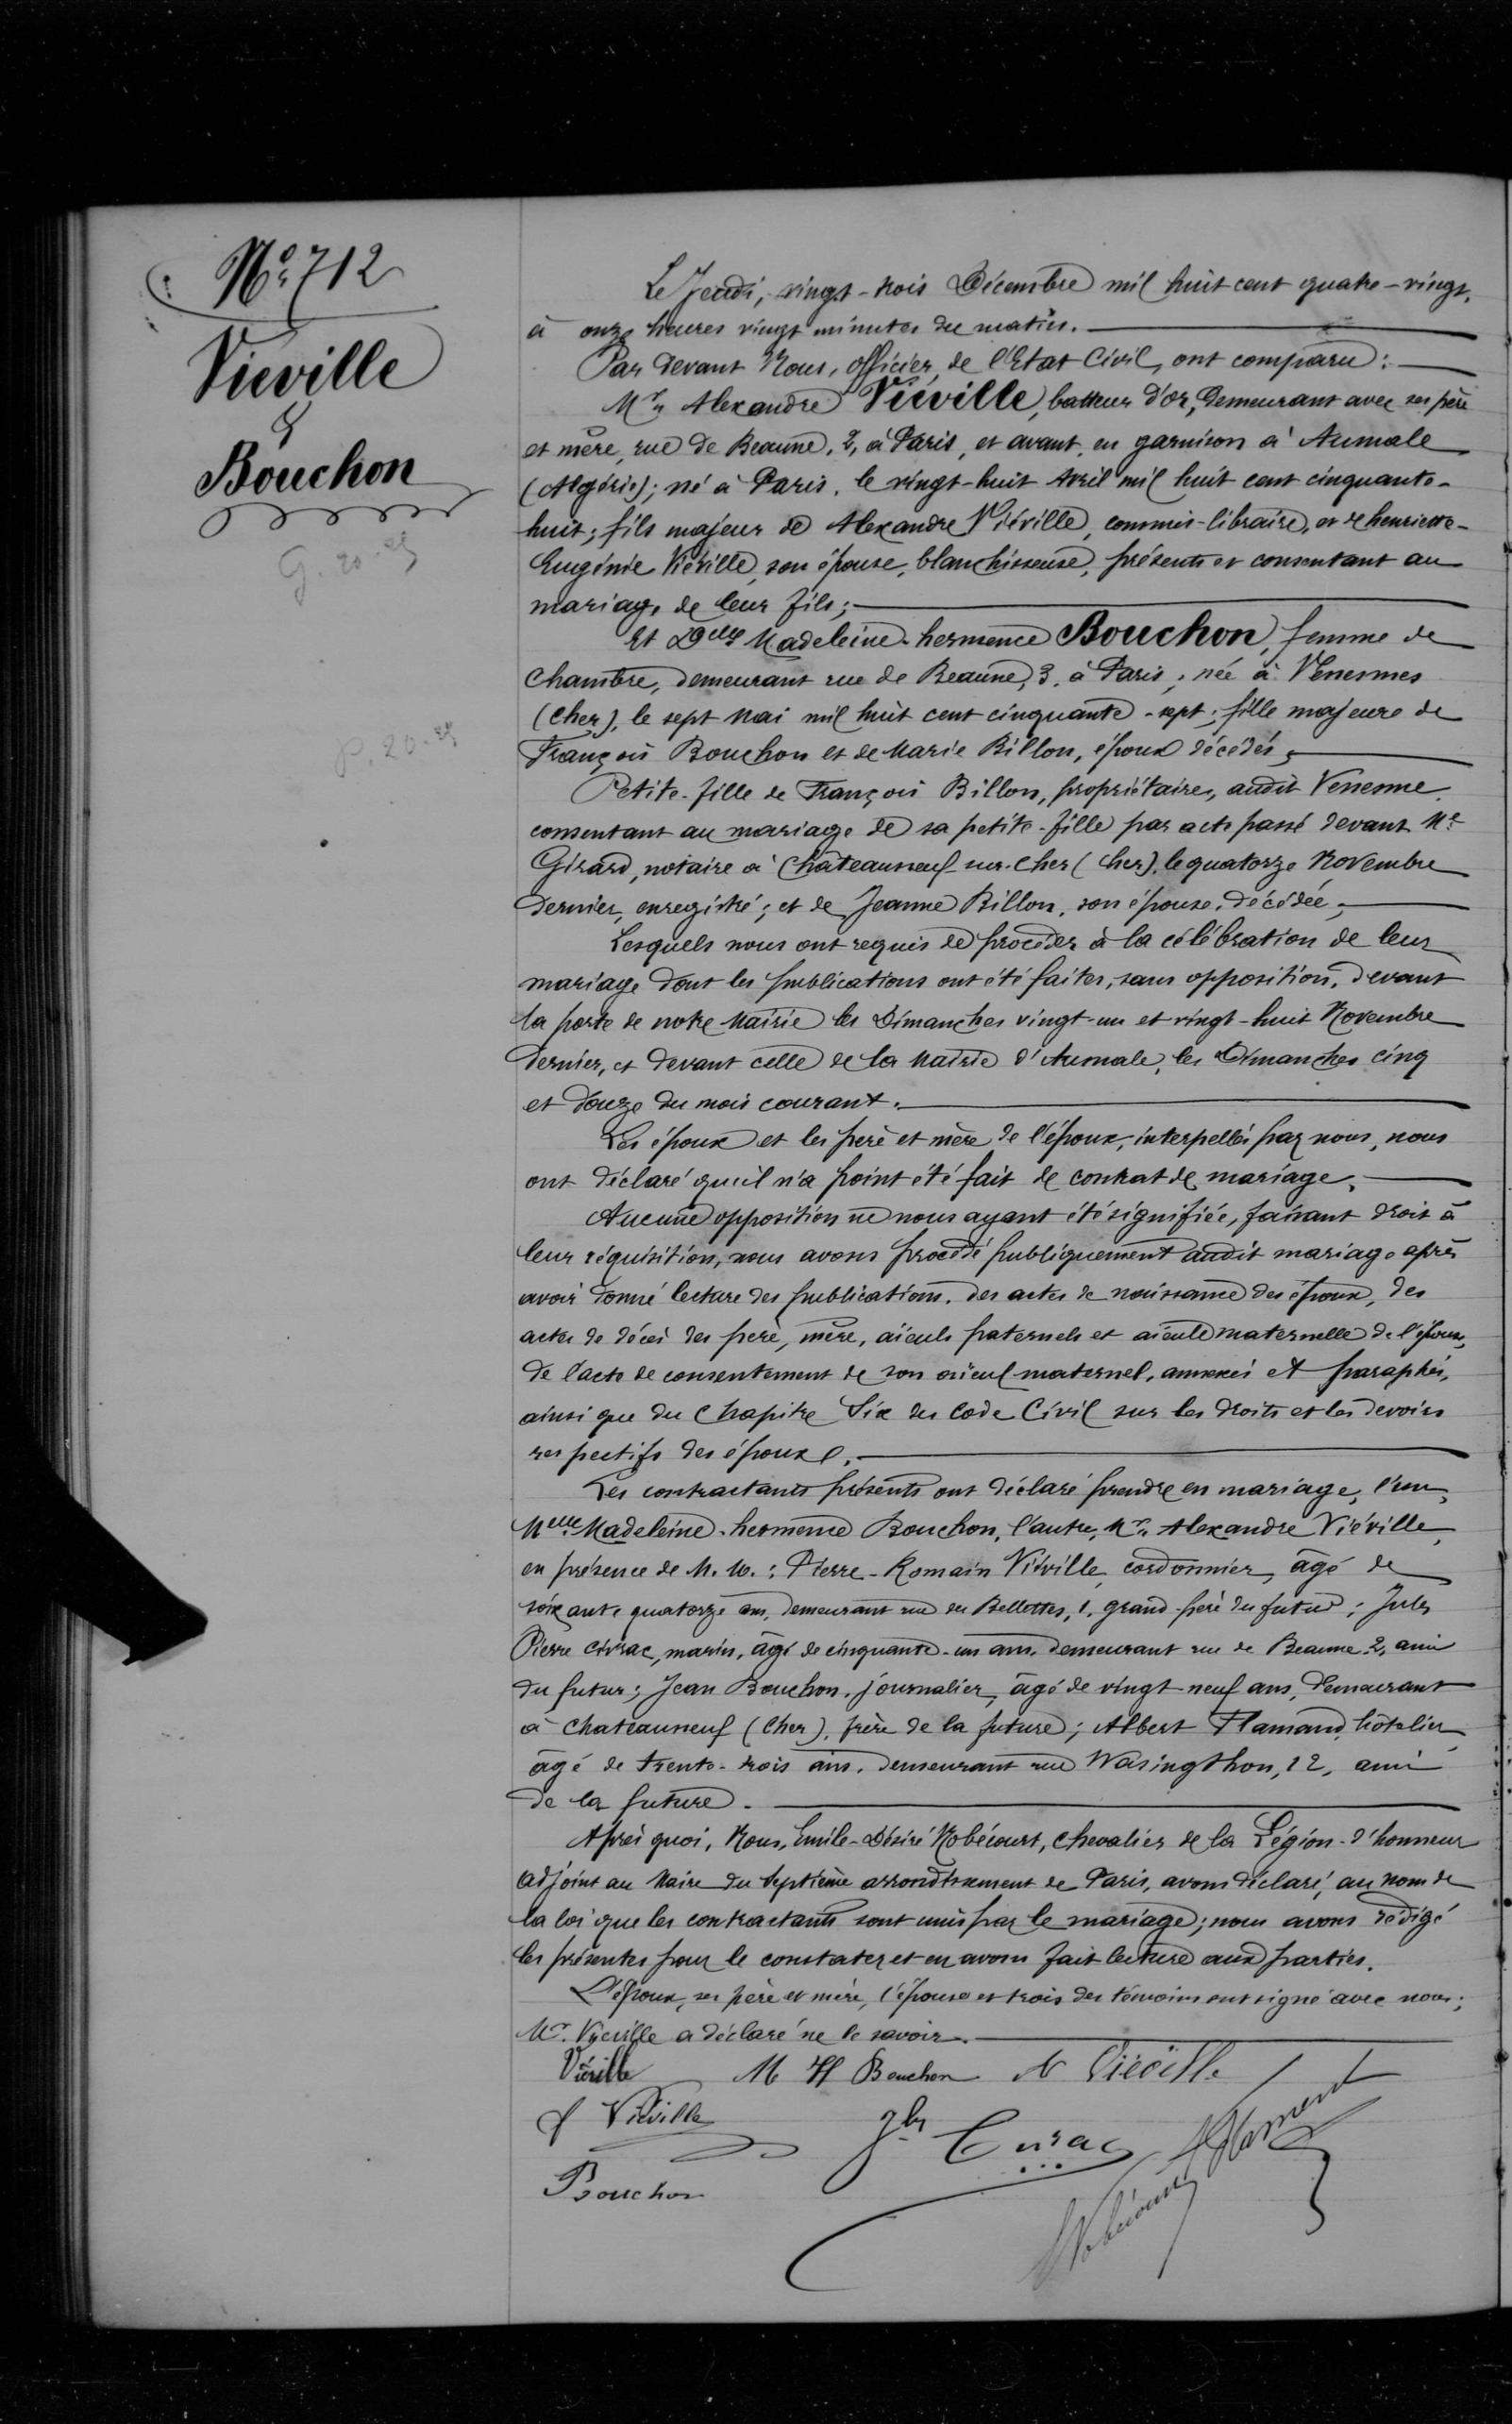N°712
Vieville
&
Bouchon
Le Jeudi, vingt-trois Décémbre mil huit cent quatre-vingt,
à onze heures vingt minutes du matin.
Par Devant Nous, officier de l'Etat Civil, ont comparu:
Me Alexandre Vieville, batteur d'or, demeurant avec ses père
et mère, rue de Beaume, 2, à Paris, et avant, en garnison à Aumale
(Algérie); né à Paris, le vingt-huit Avril mil huit cent cinquante-
huit; fils majeur de Alexandre Vieville, commis-libraire, et de Henriette-
Eugénie Vieville, son épouse, blanchisseuse, présente et consentant au
mariage, de leur fils;
Et Delle Madeleine Hermence Bouchon, femme de
chambre, demeurant rue de Beaume, 3, à Paris; née à Venesmes
(Cher), le sept mai mil huit cent cinquante-sept; fille majeure de
François Bouchon et de Marie Billon, époux décédés
Petite-fille de François Billon, propriétaire, audit Venesme
consentant au mariage de sa petite-fille par acte passé devant Me
Girard, notaire à Chateauneuf-sur-Cher (Cher) le quatorze novembre
dernier, enregistré; et de Jeanne Billon, son épouse, décédée,
Lesquels nous ont requis de procéder à la célébration de leur
mariage dont les publications ont été faites sans opposition, devant
sa porte de notre mairie les Dimanches vingt un et vingt huit novembre
dernier, et devant celle de la Mairie d'Aumale, les Dimanches cinq
et douze des mois courant.
Les époux et les père et mère de l'époux, interpellés par nous, nous
ont déclaré qu'il n'a point été fait de contrat de mariage.
Aucune opposition ne nous ayant été signifiée, faisant droit à
leur réquisition, nous avons procédé publiquement audit mariage après
avoir donné lecture des publications des actes de naissance des époux, des
actes de décès des père, mère, aïeuls paternels et aieule maternelle de l'épouse
de l'acte de consentement de son aïeu maternel, annexés et paraphés,
ainsi que du Chapitre six des Code Civil sur les droits et les devoirs
respectifs des époux.
Les contractants présents ont déclaré prendre en mariage, l'un,
Melle Madeleine Hermeme Bouchon, l'autre Mr Alexandre Viéville
en présence de M.M: Pierre Romain Viéville, cordonnier, âgé de
soixante quatorze ans, demeurant rue des Bellettes, 1, grand-frère des futurs: Jules
Pierre Cussac, marin, âgé de cinquante-un an, demeurant rue de Beaume, 2, ami
du futur; Jean Bouchon, journalier, âgé de vingt-neuf ans, demeurant
à Chateauneuf (Cher), père de la future; Albert Flamand hôtelier
âgé de trente-trois ans, demeurant rue Wasingthon, 22, ami
de la future.
Après quoi, Nous, Emile-Désirée Nobécourt, Chevalier de la Légion d'honneur
adjoint au Maire du septième arrondissement de Paris, avons déclaré, au nom de
la loi que les contractants sont unis par le mariage; nous avons rédigé
les présenter pour le constater et nous avons fait lecture aux parties.
L'époux, son père et mère, l'épouse et trois des témoins ont signé avec nous,
Me Vieville a déclaré ne le savoir.
Viéville M ll Bouchon M Viéville
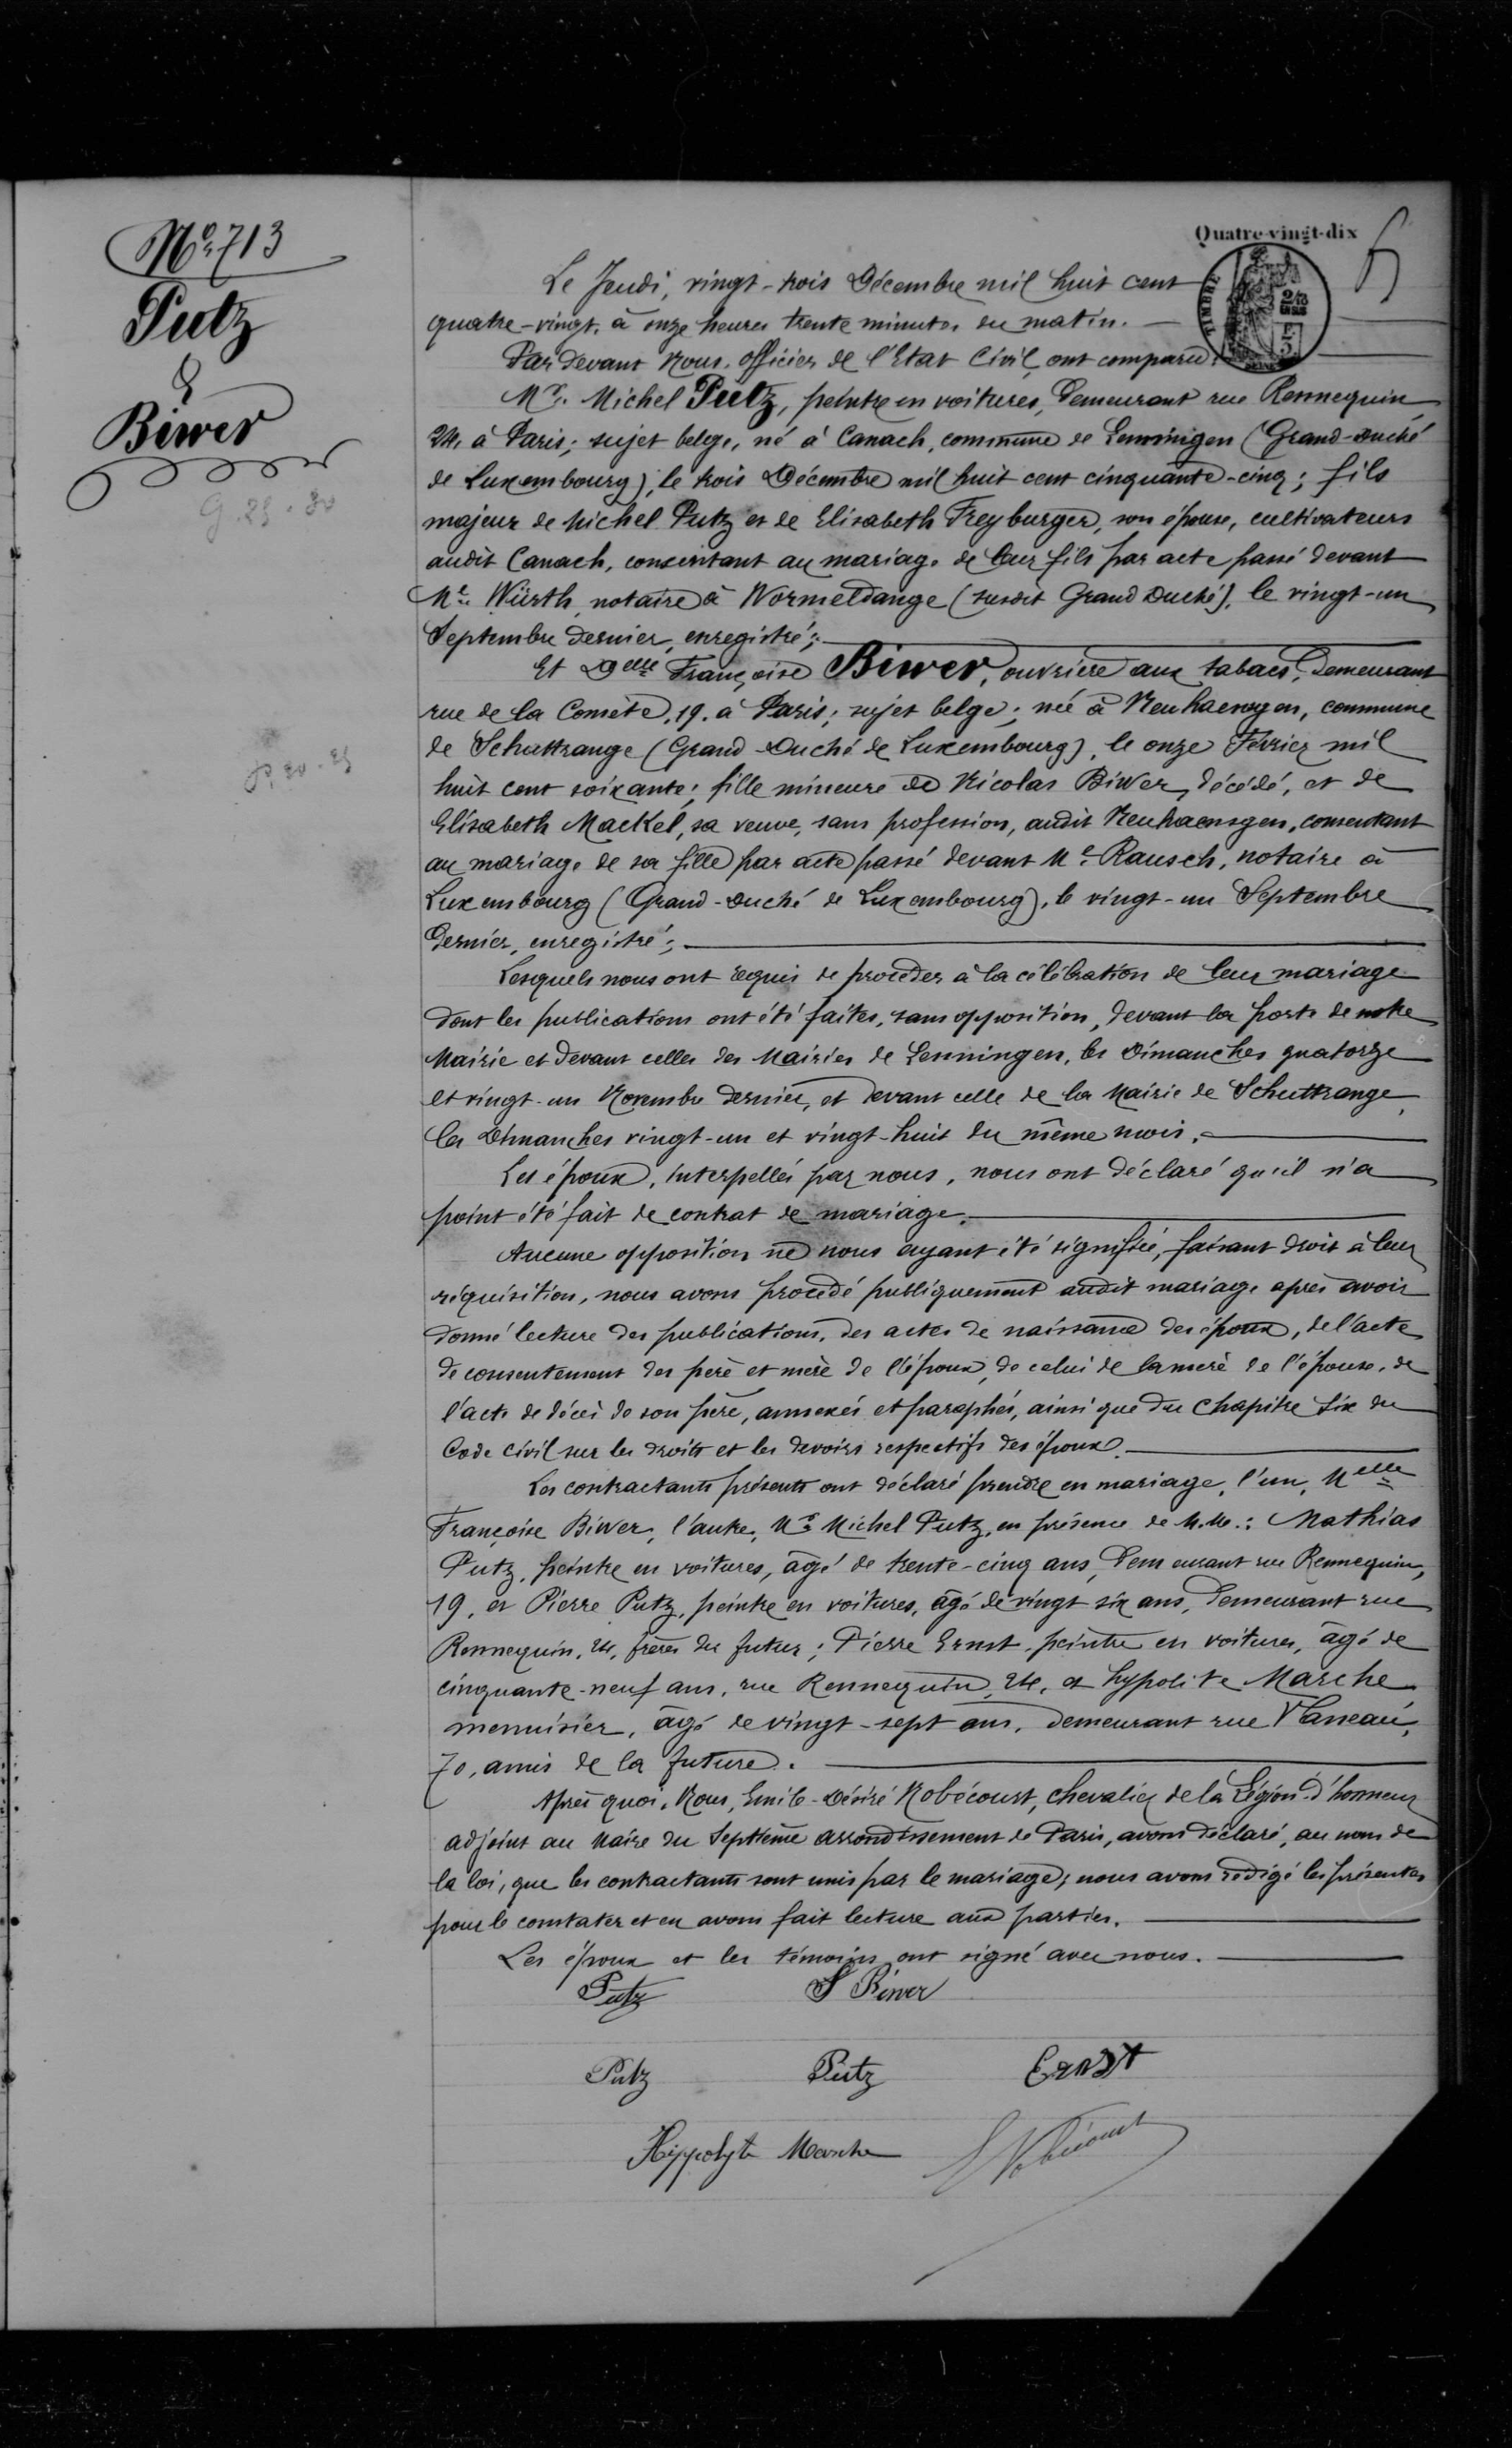N° 713
Putz
&
Biver
Le Jeudi, vingt-trois Décembre mil huit cent
quatre-vingt, à onze heures trente minutes du matin.
Pardevant Nous, officier de l'Etat Civil ont comparu:
Me Michel Peetz, peintre en voitures, demeurant rue Ronnequin
24 à Paris; sujet belge, né à Canach, commune de Lemmingen (Grand-duché
du Luxembourg) Le trois Déembre mil huit cent cinquante-cinq; fils
majeur de Michel Peetz et de Elisabeth Freyburger, son épouse, cultivateurs
audit Canach, consentant au mariage de leur fils par acte passé devant
Me Würth, notaire à Wormeldance (susdit Grand Duché) le vingt-un
septembre dernier enregistré:
Et Delle Françoise Bisver, ouvrière aux tabacs, demeurant
rue de la Comète, 19, à Paris, sujet belge; ne à Neuhaenogen, commune
de Sehattrange (Grand Duché de Luxembourg), le onze Février mil
huit cent soixante; fille mineure de Nicolas Biwer, décédé, et de
Elisabetch Mackel sa veuve, sans profession, audit Neuhaenogen, consentant
au mariage de sa fille par acte passé devant Me Rausch, notaire à
Luxembourg (Grand Duché de Luxembourg) le vingt-un Septembre
dernier, enregistré.
Lesquels nous ont requis de procéder à la célébration de leur mariage
dont les publications ont été faites, sans opposition, devant la poste de notre
mairie et devant celle des mairies de Lessningen, les Dimanches quatorze
et vingt-un Novembre dernier, et devant celle de la Mairie de Schuttrange
les dimanches vingt-un et vingt-huit du même mois.
Les époux, interpellés par nous, nous ont déclaré qu'il n'a
point été fait de contrat de mariage
Aucune opposition ne nous ayant été signifié, faisant droit à leur
réquisition, nous avons procédé publiquement audit mariage après avoir
donné lecture des publications, des actes de naissance des époux, de l'acte
de consentement des père et mère de l'époux, de celui de la mère de l'épouse, de
l'acte de décès de son père, annexés et paraphées, ainsi que du chapitre six des
Code Civil sur les droits et les devoirs respectifs des époux.
Les contractants présents ont déclaré prendre en mariage, l'un, Melle
Françoise Biwer, l'autre, M. Michel Peetz, en présence de Mlle: Mathias
Peetz, peintre en voiture, âgé de trente-cinq ans, demeurant rue Rennequin,
19, et Pierre Peetz, peintre en voiture, âgé de vingt-six ans, demeurant rue
Rennequin, 24, frères du futurs: Pierre Ernest, peintre en voitures, âgé de
cinquante-neuf ans, rue Rennequin, 24, et hypocrite Marche
menuisier, âgé de vingt-sept ans, demeurant rue Vlaneauu,
70, amis de la future.
Après quoi, Nous, Emile-Désiré Robécourt, chevalier de la Légion d'Honneur
adjoint au Maire du septième arrondissement de Paris, avons déclaré, au nom de
la loi, que les contractants sont unis par le mariage: nous avons rédigé les présentes
pour le constater et en avons fait lecture aux parties.
Les époux et les témoins ont signé avec nous